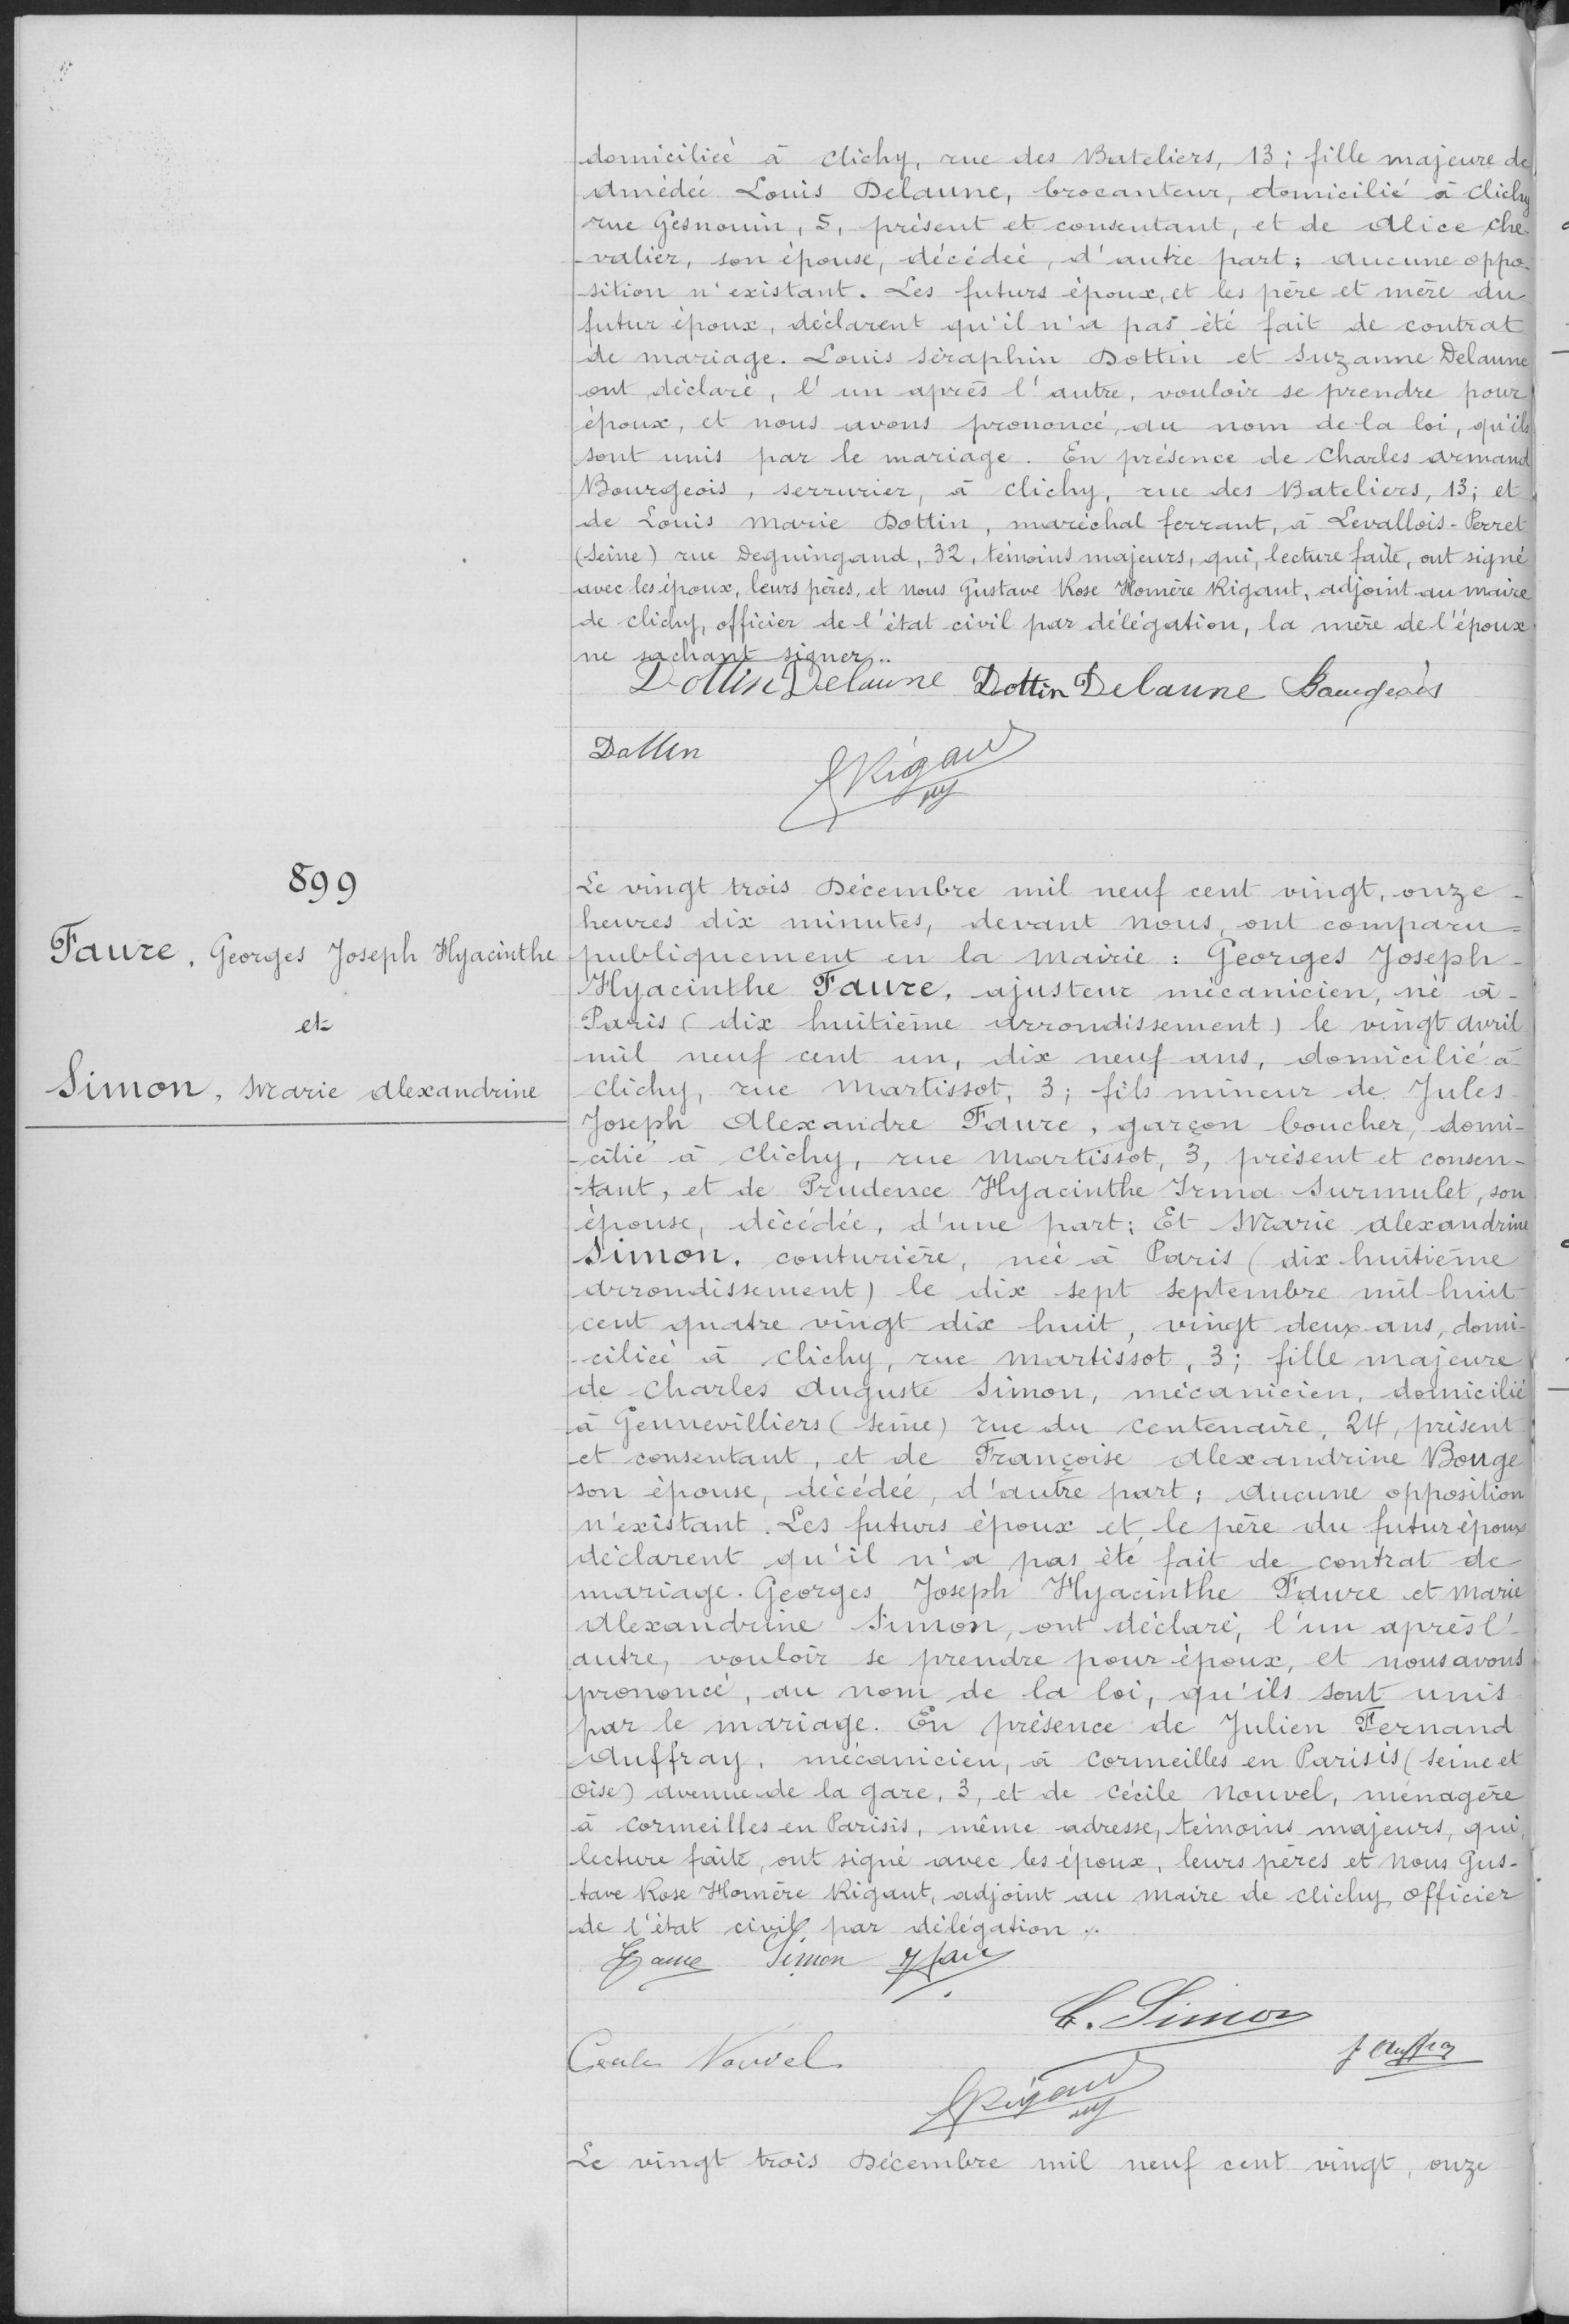domiciliée à Clichy, rue des Bateliers, 13; fille majeure de
Amédée Louis Delaune, brocanteur, domicilié à Clichy
rue Gesnouin, 5, présent et consentant, et de Alice, che-
valier, son épouse, décédée, d'autre part; aucune oppo-
sition n'existant. Les futurs époux et les père et mère du
futur époux, déclarent qu'il n'a pas été fait de contrat
de mariage. Louis Séraphin Dottin et Suzanne Delaune
ont déclaré, l'un après l'autre, vouloir se prendre pour
époux, et nous avons prononcé, au nom de la loi, qu'ils
sont unis par le mariage. En présence de Charles Armand
Bourgeois, serrurier, à Clichy, rue des Bateliers, 13; et
de Louis Marie Dottin, maréchal ferrant, à Levallois-Perret
(Seine) rue Deguingand, 32, témoins majeurs, qui, lecture faite, ont signé
avec les époux, leurs pères, et Nous Gustave Rose Homère Rigaut, adjoint au maire
de clichy, officier de l'état civil par délégation, la mère de l'époux
ne sachant signer.
899
Faure, Georges Joseph Hyacinthe
et
Simon, Marie Alexandrine
Le vingt trois Décembre mil neuf cent vingt, onze
heures dix minutes, devant nous ont comparu
publiquement en la mairie: Georges Joseph
Hyacinthe Faure, ajusteur mécanicien, né à
Paris (dix huitième arrondissement) le vingt avril
mil neuf cent un, dix neuf ans, domicilié à
clichy, rue Martissot, 3. fils mineur de Jules
Joseph Alexandre Faure, garçon boucher, domi
-cilié à clichy, rue Martissot, 3, présent et consen
-tant, et de Prudence Hyacinthe Irma Surmilet, son
épouse, décédée, d'une part: Et Marie Alexandrine
Simon, couturière, née à Paris (dis-huitième
arrondissement) le dix sept septembre mil huit
cent quatre vingt dix huit, vingt deux ans, domi
-ciliée à clichy, rue Martissot, 3; fille majeure
de Charles Auguste Simon, mécanicien, domicilié
à Gennevilliers (Seine) rue ru centenaire, 24, présent
et consentant, et de Françoise Alexandrine Bouge
son épouse, décédée, d'autre part: Aucune opposition
n'existant. Les futurs époux et le père du futur époux
déclarent qu'il n'a pas été fait de contrat de
mariage. Georges Joseph Hyacinthe Faure et Marie
Alexandrine Simon, ont déclaré, l'un après l'
autre, vouloir se prendre pour époux, et nous avons
prononcé, au nom de la loi, qu'ils sont unis
par le mariage. En présence de Julien Fernand
Auffray, mécanicien, à Cormeilles en Parisis (Seine et
Oise) avenue de la gare, 3, et de Cécile nouvel, ménagère
à Cormeilles en Parisis, même adresse, témoins majeurs, qui
lecture faite, ont signé avec les époux, leurs pères et nous Gus-
-tave Rose Homère rigaut, adjoint au maire de clichy, officier
de l'état civil par délégation ./.
Le vingt trois Décembre mil neuf cent vingt, onze
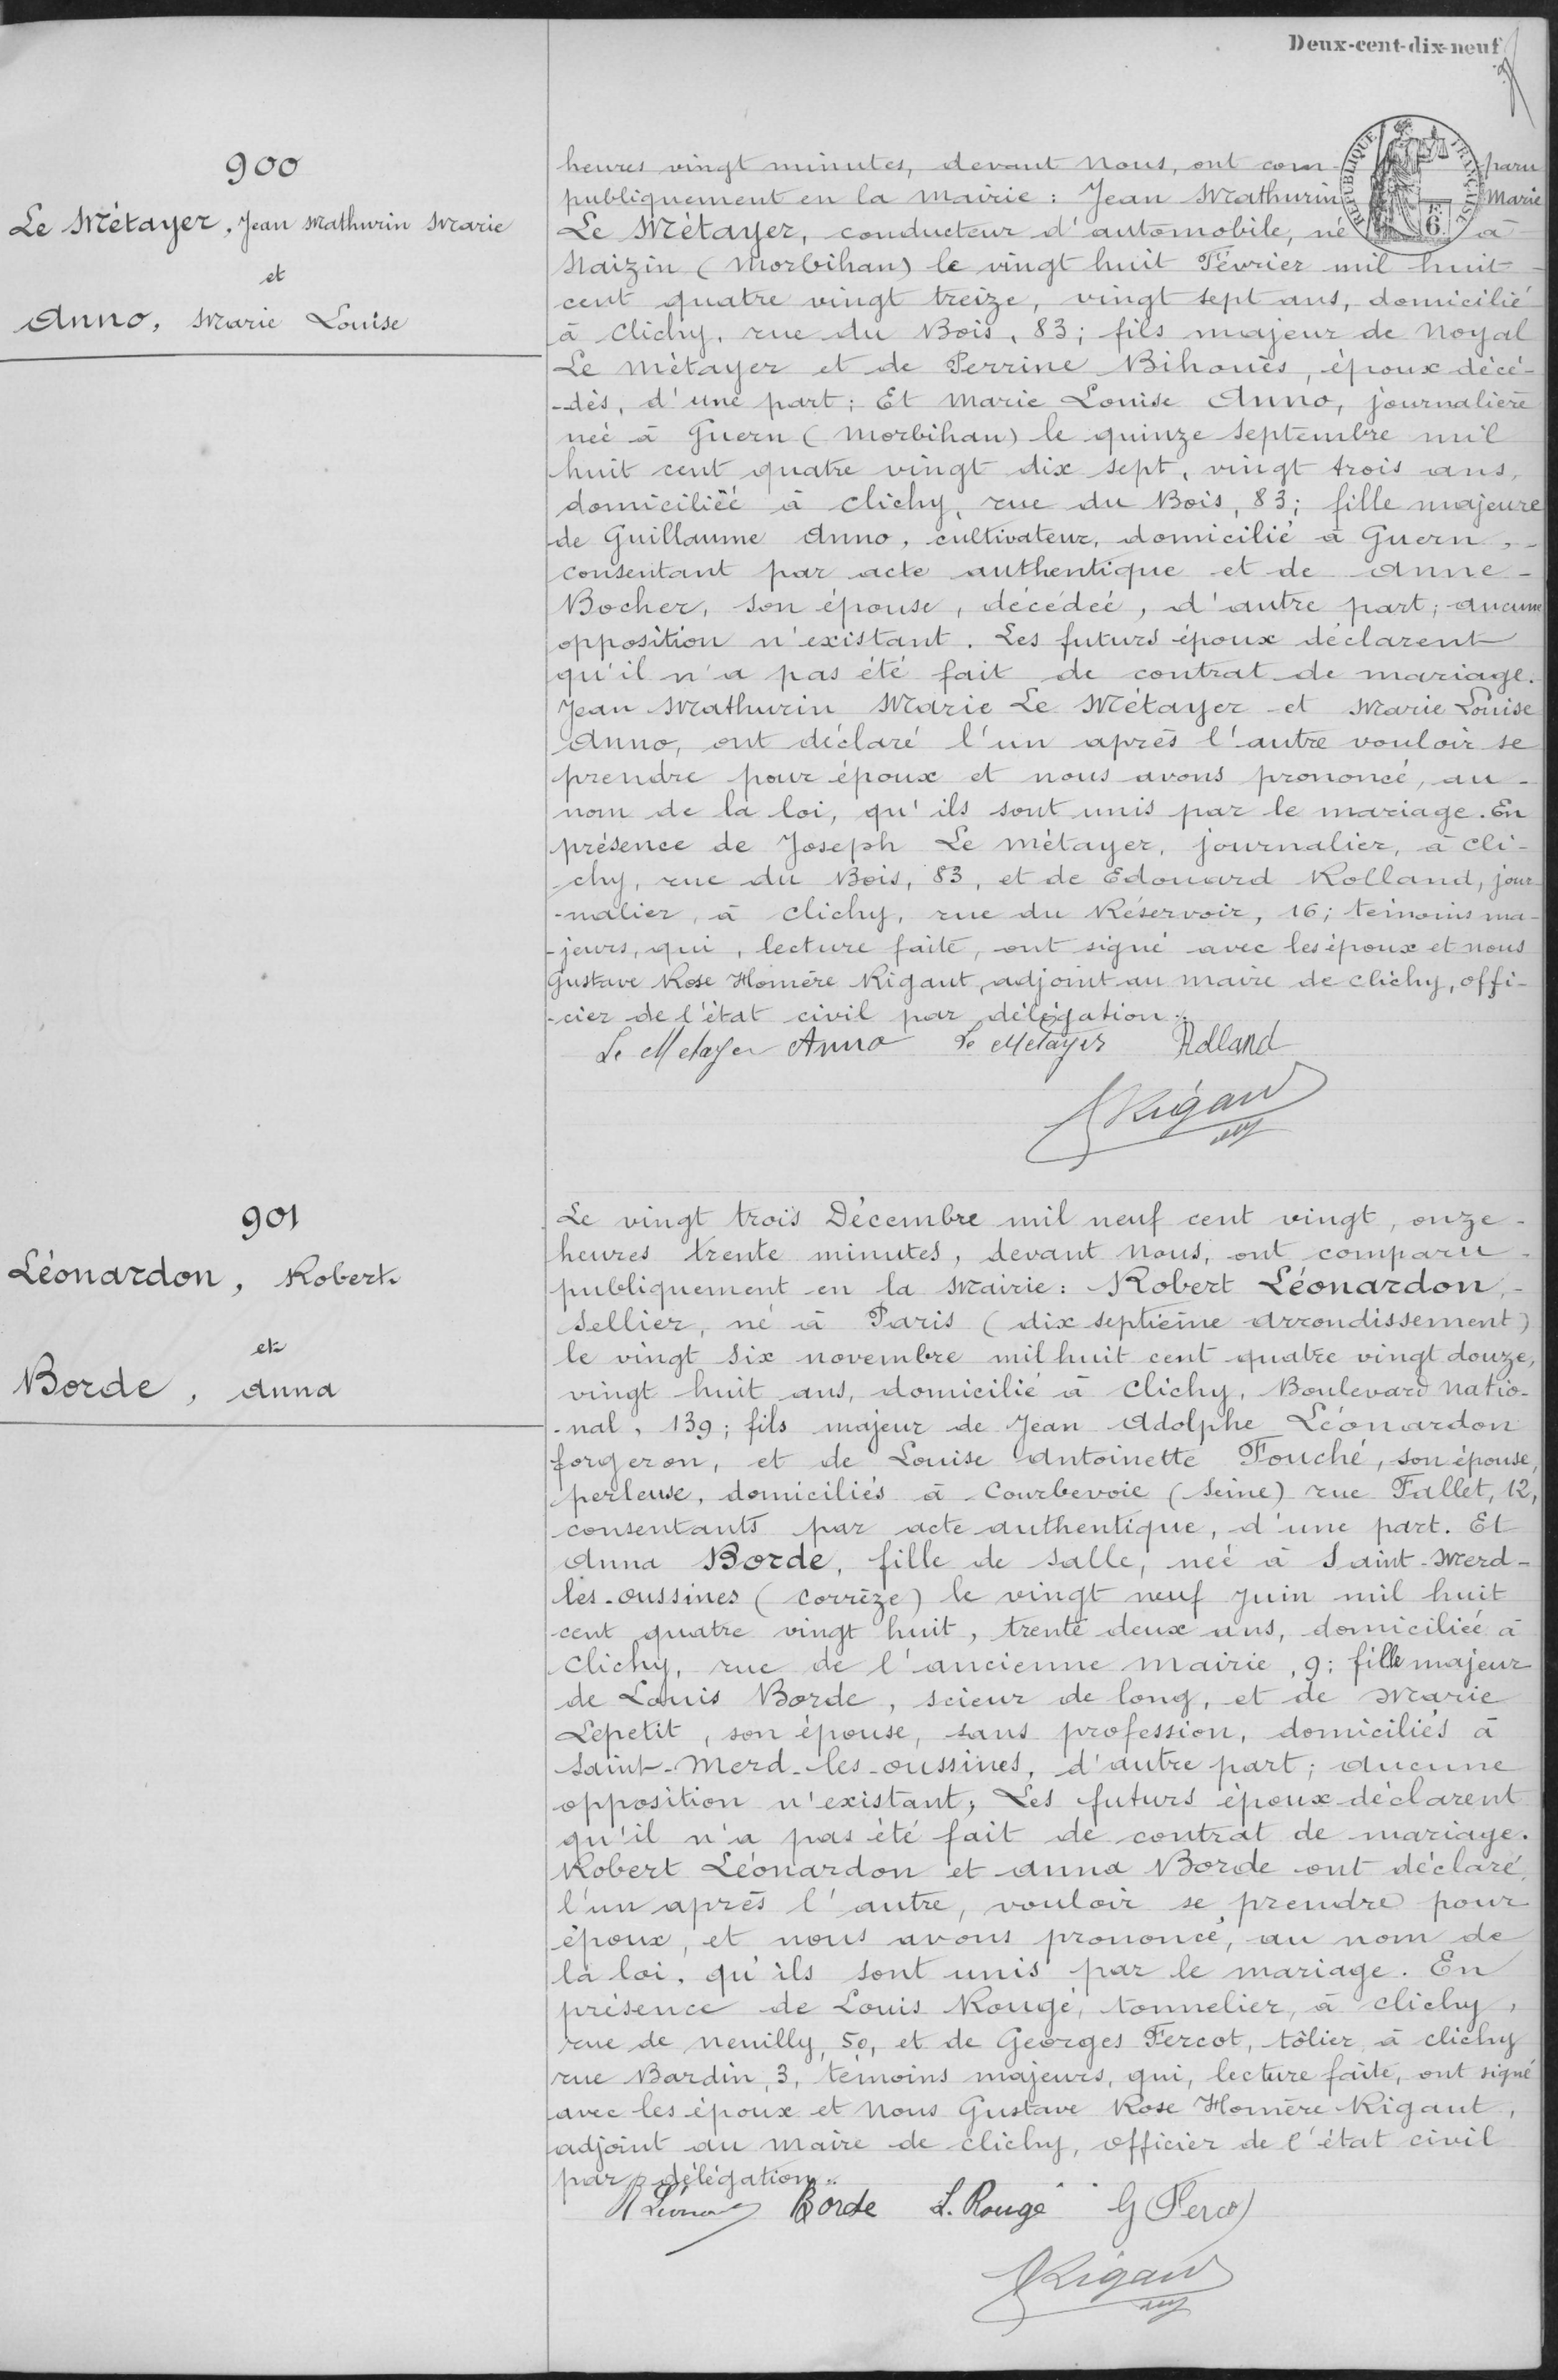900
Le Métayer, Jean Mathurin Marie
et
Anno, Marie Louise
heures vingt minutes, devant Nous ont comparu
publiquement en la Mairie: Jean Mathurin Marie
Le Métayer, conducteur d'automobile, né à
Maizin (Morbihan) le vingt-huit Février mil huit
cent quatre vingt treize, vingt sept ans, domicilié
à Clichy, rue du Bois, 83: fils majeur de Noyal
Le Métayer et de Perrine Bihouès, époux décé-
dés, d'une part: Et Marie Louise Anno, journalière
née à Guern (Morbihan) le quinze septembre mil
huit cent quatre vingt dix sept, vingt trois ans
domiciliée à Clichy, rue du Bois, 83: fille majeure
de Guillaume Anno, cultivateur, domicilié à Guern,
consentant par acte authentique et de Anne
Bocher, son épouse, décédée, d'autre part; aucune
opposition n'existant. Les futurs époux déclarent
qu'il n'a pas été fait de contrat de mariage.
Jean Mathurin Marie Le Métayer et Marie Louise
Anno, ont déclaré l'un après l'autre vouloir se
prendre pour époux et nous avons prononcé, au
nom de la loi, qu'ils sont unis par le mariage. En
présence de Joseph Le Métayer, journalier à cli-
chy, rue du Bois, 83, et de Edouard Rolland, jour
-nalier à clichy, rue du Réservoir, 16; témoins ma
-jeurs, qui, lecture faite, ont signé avec les époux et nous
Gustave Rose Homère Rigaut, adjoint au maire de clichy, offi-
-cier de l'état civil par délégation ./.
901
Léonardon, Robert
et
Borde, Anna
Le vingt trois Décembre mil neuf cent vingt, onze
heures trente minutes, devant nous, ont comparu
publiquement en la Mairie: Robert Léonardon
Sellier, né à Paris (dix septième arrondissement)
le vingt six novembre mil huit cent quatre vingt douze
vingt huit ans, domicilié à clichy, Boulevard natio-
-nal, 139; fils majeur de Jean Adolphe Léonardon
forgeron, et de Louise Antoinette Fouché, son épouse,
perleuse, domiciliés à Courbevoie (Seine) rue Fallet, 12,
consentants par acte authentique, d'une part. Et
Anna Borde, fille de salle, née à Saint Werd-
les-Oussines (Corrèze) le vingt neuf juin mil huit
cent quatre vingt huit, trente deux ans, domiciliée à
Clichy, rue de l'ancienne mairie, 9: fille majeur
de Louis Borde, scieur de long, et de Marie
Lepetit, son épouse, sans profession, domiciliés à
Saint-Merd-les-Oussines, d'autre part; aucune
opposition n'existant. Les futurs époux déclarent
qu'il n'a pas été fait de contrat de mariage.
Robert Léonardon et Anna Borde ont déclaré
l'un après l'autre, vouloir se prendre pour
époux, et nous avons prononcé, au nom de
la loi, qu'ils sont unis par le mariage. En
présence de Louis Rougé, tonnelier, à clichy,
rue de Neuilly, 50, et de Georges Fercot, tôlier à Clichy
rue Bardin, 3, témoins majeurs, qui, lecture faite, ont signé
avec les époux et nous Gustave Rose Homère-Rigaut,
adjoint au maire de clichy, officier de l'état civil
par délégation